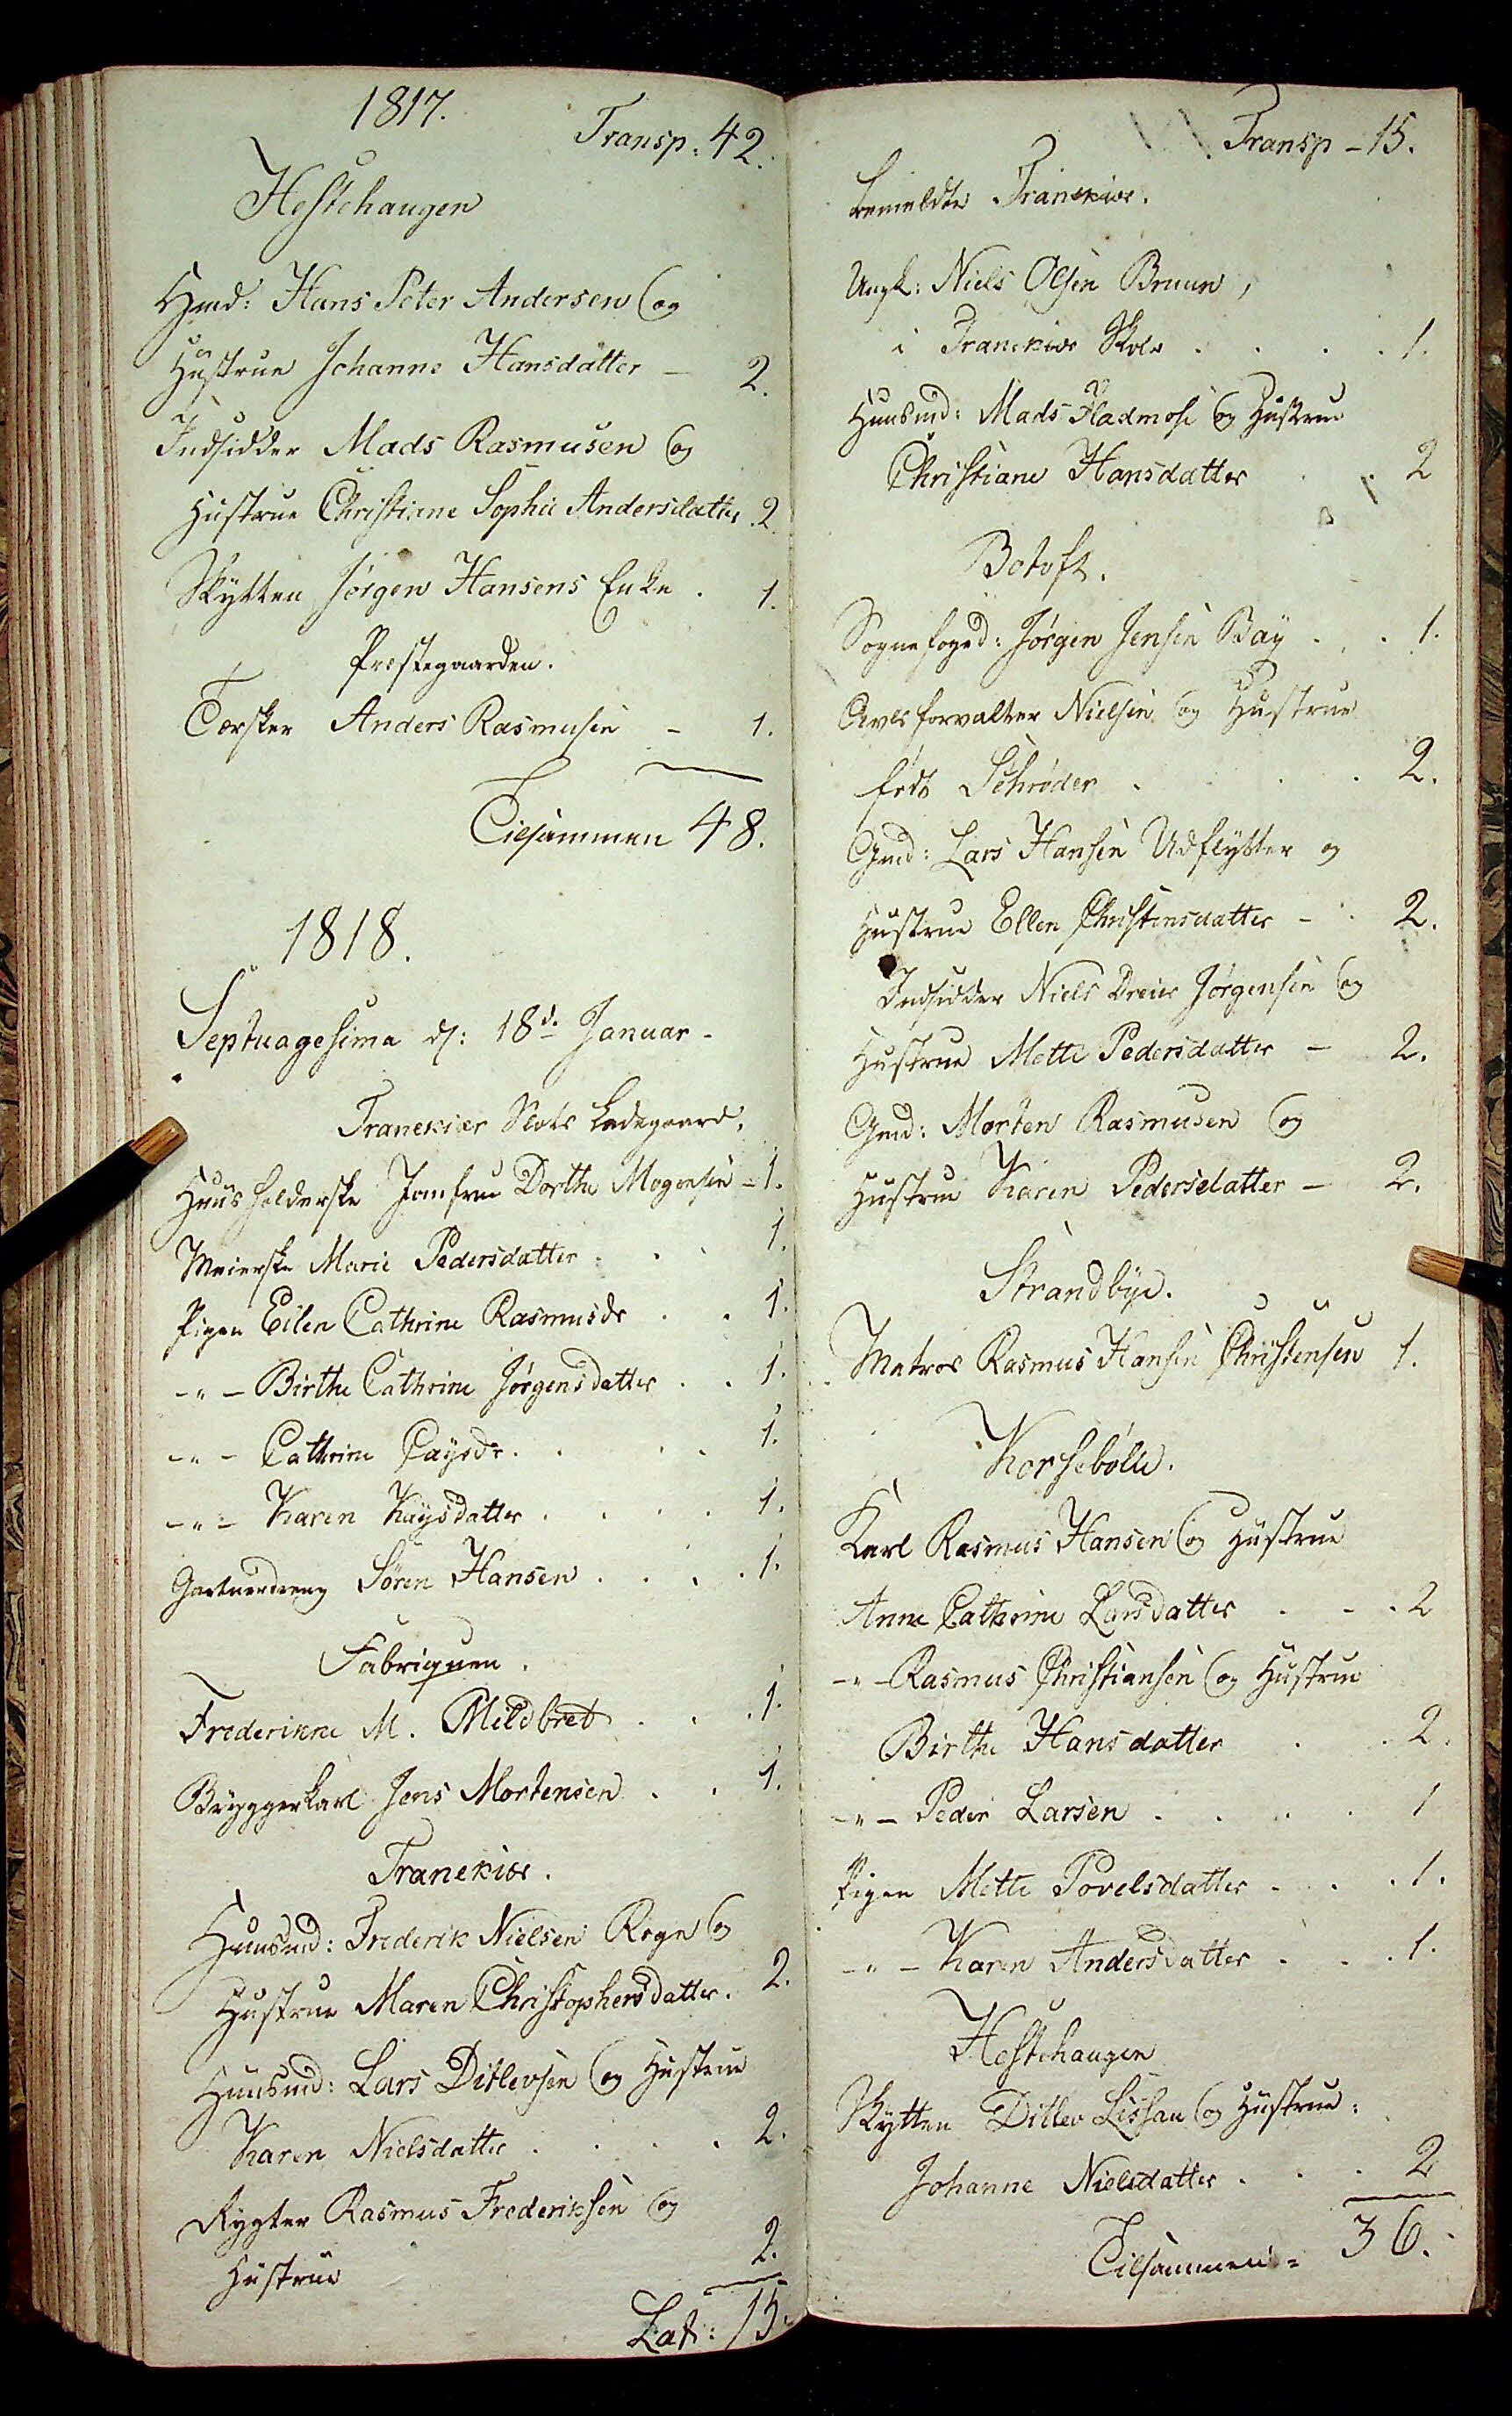1817.
Transp: 42.
Hestehaugen
Hmd: Hans Peter Andersen og
Hustrue Johanne Hansdatter - 2.
Indsidder Mads Rasmusen og
Hustrue Christiane Sophie Andersdatter . 2.
Skytten Jørgen Hansens Enke . 1.
Præstegaarden.
Tærskerr Anders Rasmusen - 1.
Tilsammen 48.
1818
Septuagesima d: 18de Januar.
Tranekiær Slots Ladegaard.
Huusholderske Jomfrue Dorthe Mogensen - 1.
Meierske Marie Pedersdatter . . . 1.
Pigen Ellen Cathrine Rasmusdr . . 1.
-"- Birthe Cathrine Jørgensdatter . . 1.
-"- Cathrine Caysdr . . . . 1.
-"- Karen Kaysdatter . . . . 1.
Gartnerdreng Søren Hansen . . . . 1.
Fabriquen
Friderikke M. Mildbret . . . 1.
Bryggerkarl Jens Mortensen . . 1.
Tranekiær.
Huusmd: Friderik Nielsen Rogn og
Hustrue Maren Christophersdatter . 2.
Huusmd: Lars Ditlevsen og Hustrue
Karen Nielsdatter . . . . 2.
Rygter Rasmus Frederiksen og
Hustrue 2.
Lat: 15
Transp - 15.
bemeldte Tranekiær.
Ungk: Niels Olsen Bruun,
i Tranekiær Skole . . . . 1.
Huusmd: Mads Fladmose og Hustrue
Christian Hansdatter . . 2
Botoft.
Sognefoged: Jørgen Jensen Bay . . 1.
Avlsforvalter Nielsen og Hustrue
fødst Schrøder . . . . 2.
Gmd: Lars Hansen Udflytter og
Hustrue Ellen Christensdatter - . 2.
Indsidder Niels Dreier Jørgensen og
Hustrue Mette Pedersdatter - 2.
Gmd: Morten Rasmusen og
Hustrue Karen Pedersdatter - 2.
Strandbye.
Matros Rasmus Hansen Christensens 1.
Korsebølle.
Karl Rasmus Hansens og Hustrue
Anne Cathrine Larsdatter . . . 2
-"- Rasmus Christiansen og Hustrue
Birthe Hansdatter . . 2.
-"- Peder Larsen . . . . 1
Pigen Mette Povelsdatter . . . 1.
-"- Karen Andersdatter . . . 1.
Hestehaugen
Skytten Ditlev Lissau og Hustrue:
Johanne Nielsdatter . . . 2.
Tilsammend = 36.
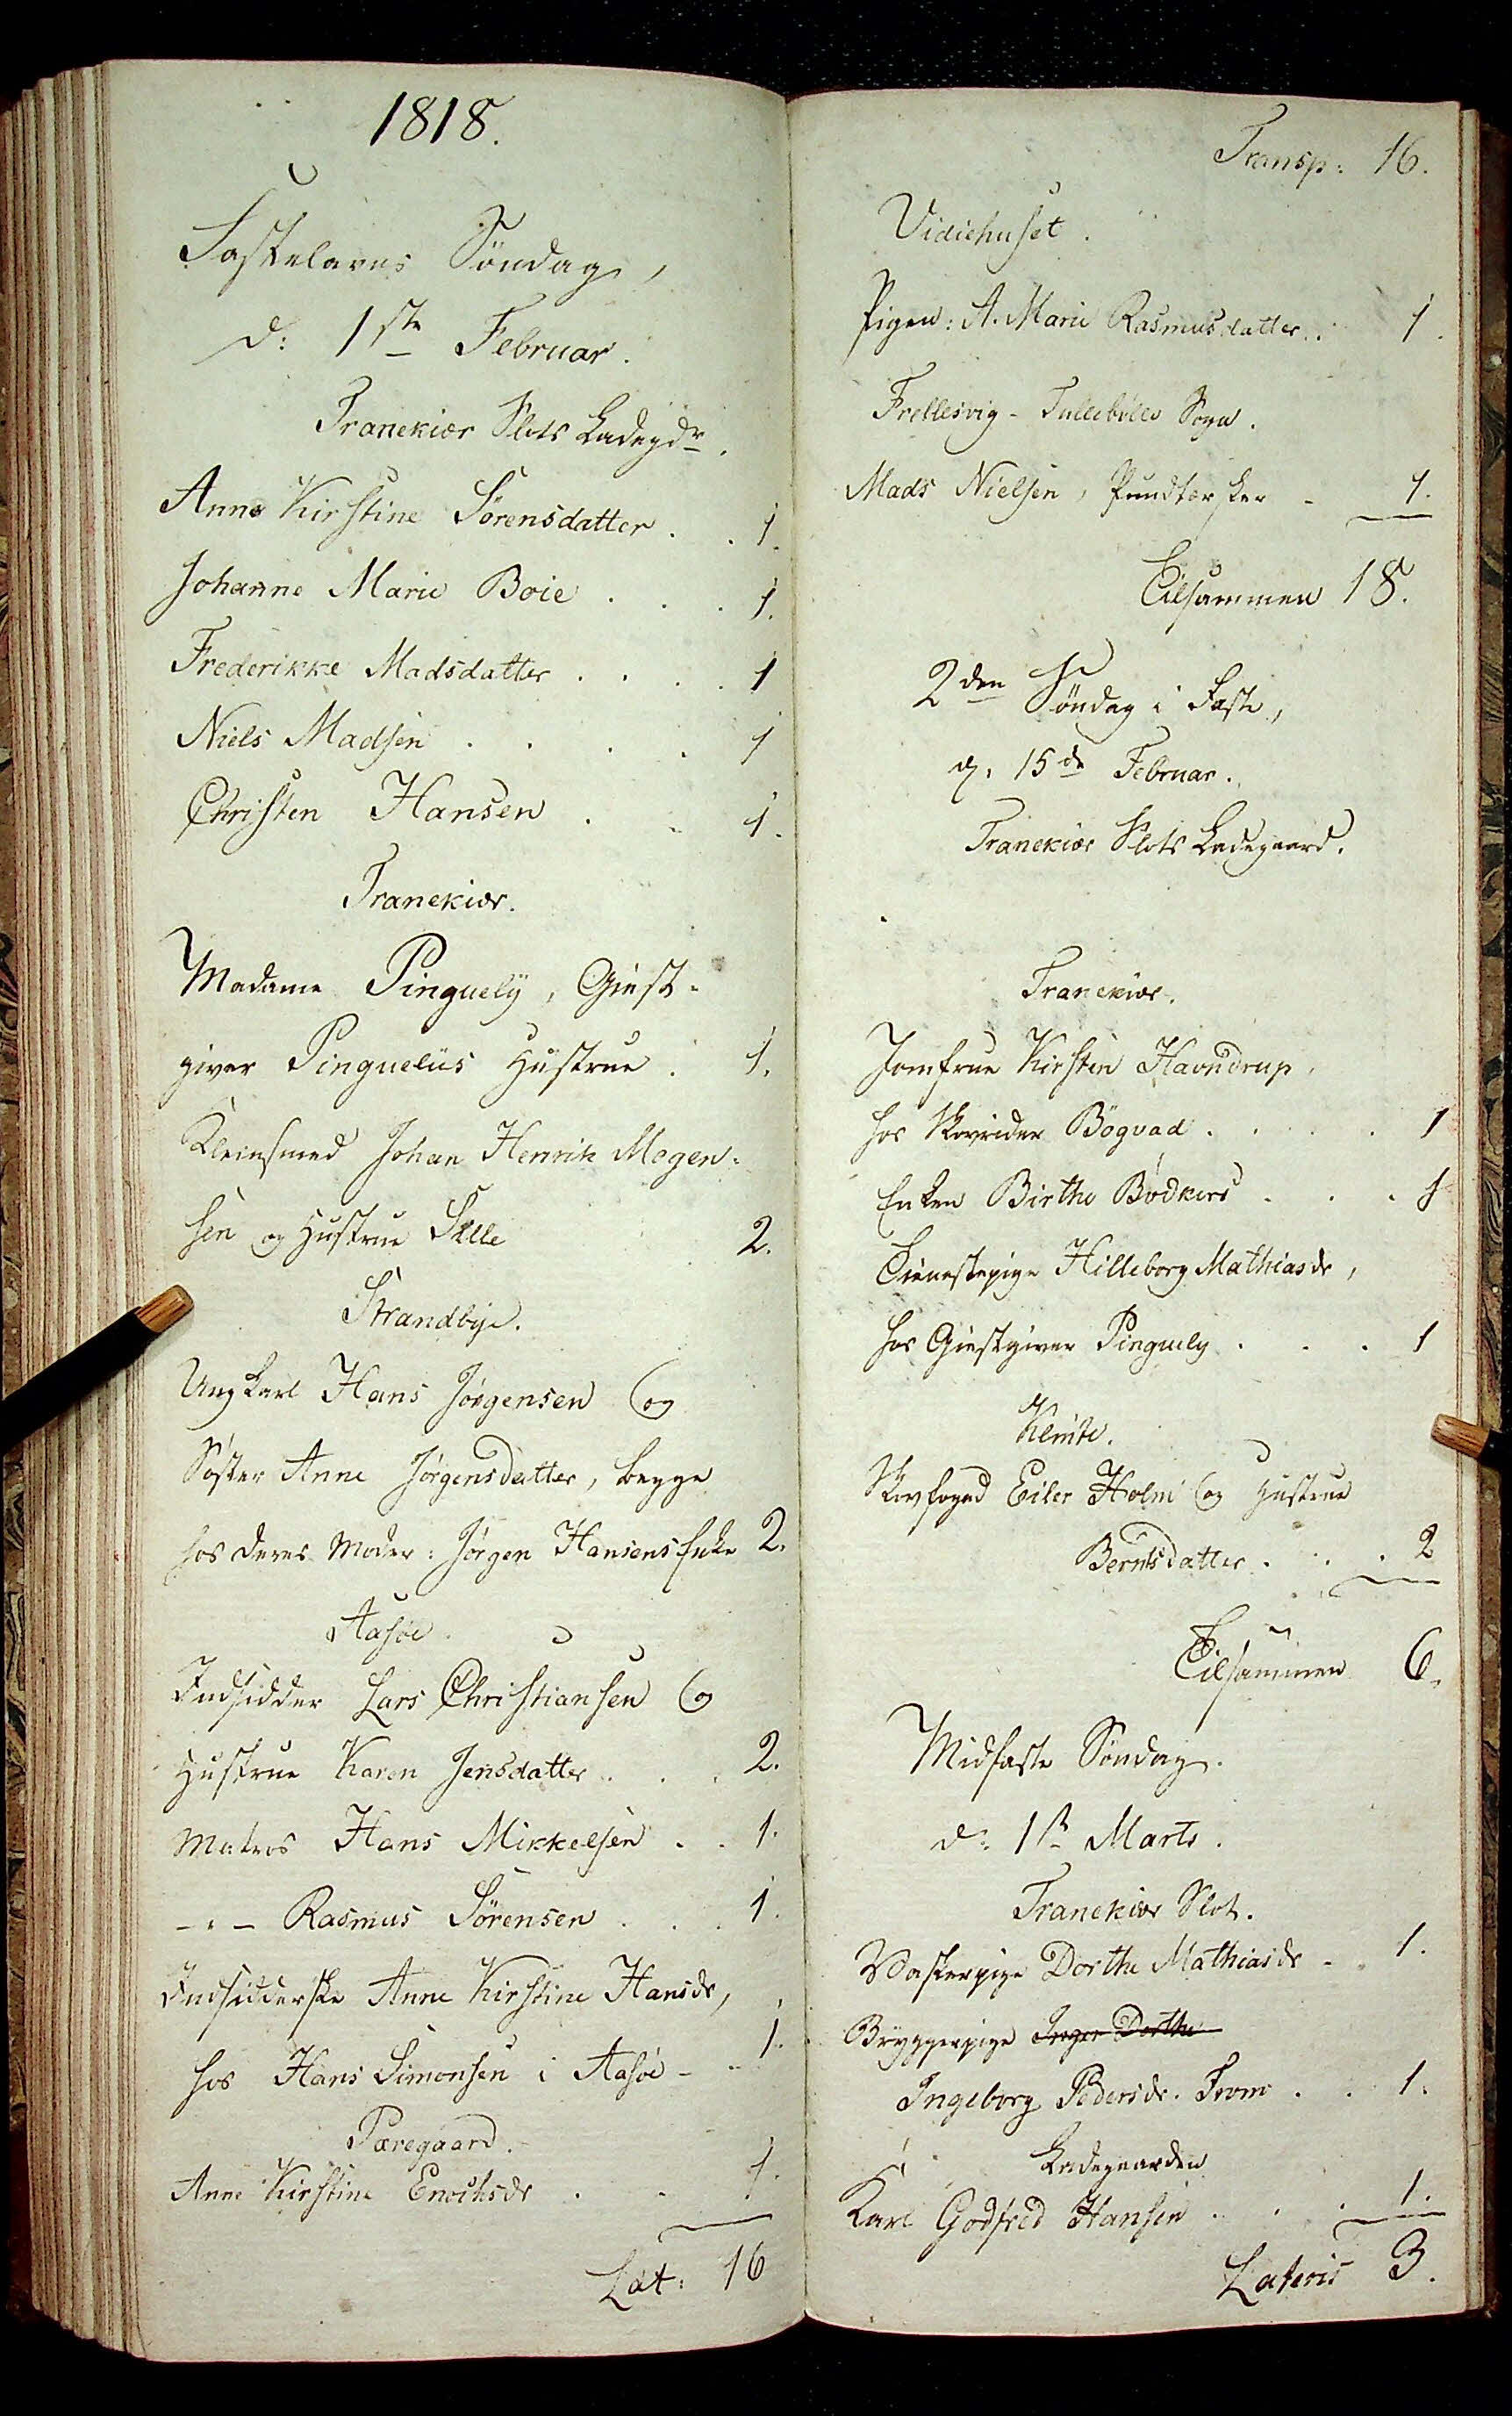1818.
Fastelavns Søndag
d. 1ste Februar.
Traneliær Slots Ladegdr
Anne Kirstine Sørensdatter . . 1.
Johanne Marie Boie . . . 1.
Frederikke Madsdatter . . . . 1.
Niels Madsen . . . . 1
Christen Hansen . .1.
Tranekiær.
Madame Pinguely,  Giest¬
giver Pinguelus Hustrue . 1.
Kleinsmed Johan Henrik Mogens¬
sen og Hustrue Sille 2.
Strandbye.
Ungkarl Hans Jørgensen og
Søster Anne Jørgensdatter, begge
hos deres Moder: Jørgen Hansens Enke 2.
Aasøe.
Indsidder Lars Christiansen og
Hustrue Karen Jensdatter . . . 2.
Matros Hans Mikkelsen . . 1.
-"- Rasmus Sørensen . . . 1.
Indsidderske Anne Kirstine Hansdr,
hos Hans Simonsen i Aasøe - - 1.
Pæregaard.
Anne Kirstine Enochsdr . .1.
Lat: 16
Transp: 16.
Vidiehuset
Pigen: A. Marie Rasmusdatter . . 1.
Frellesvig - Tullebølle Sogn.
Mads Nielsen, Pundtærsker - 1.
Tilsammen 18.
2den Søndag i Faste,
d: 15de Februar.
Tranekiær Slots Ladegaard.
Tranekiær.
Jomfrue Kirsten Havndrup.
hos Skovrider Bøgvad . . . . 1
Enken Birthe Bøkdkers . . . 1
Tienestepige Hilleborg Mathiasdr,
og Giestgiver Pinguely . . . 1
Klinte.
Skovfoged Eiler Holm og Hustrue
Berntsdatter . . . 2
Tilsammen 6.
Midfaste Søndag.
d: 1ste Marts.
Tranekiær Slot.
Vaskerpige Dorthe Mathiasdr . 1.
Bryggerpige Inger Dorthe
Ingeborg Pedersdr. From . . 1.
Ladegaarden
Karl Godfred Hansen . . . 1.
Lateris 3.